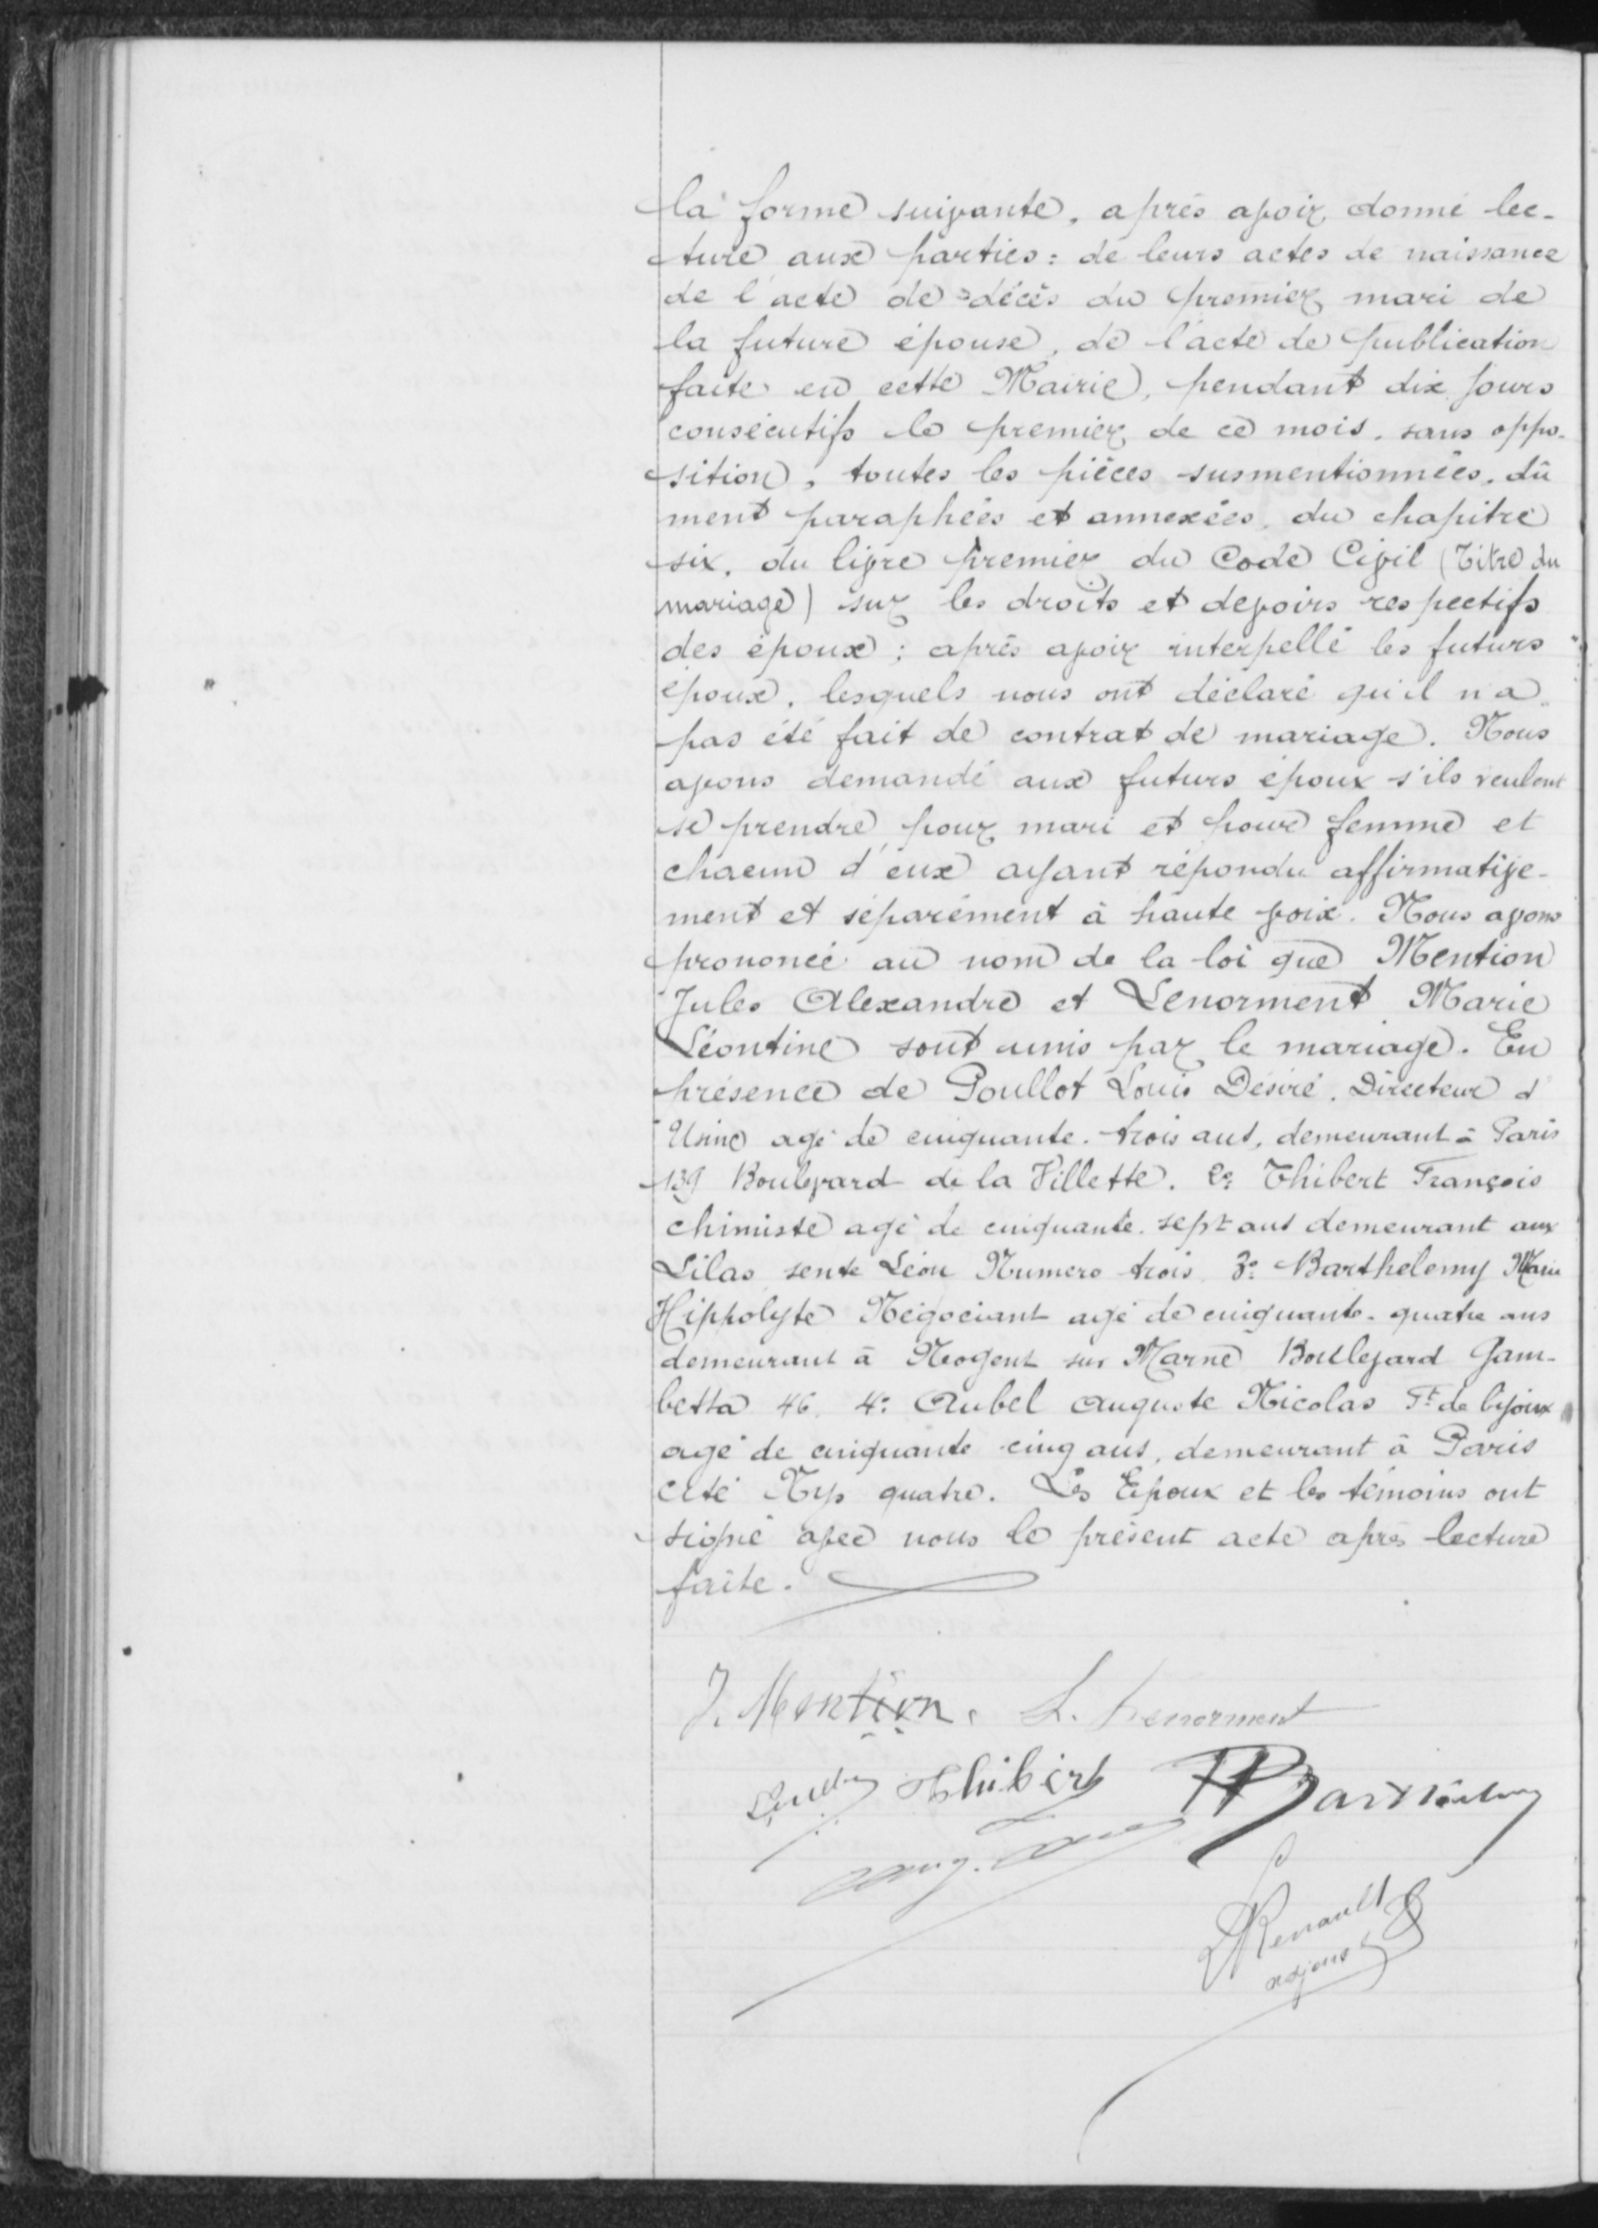la forme suivant, après avoir donné lec-
ture aux parties: de leurs actes de naissance
de l'acte de décès du premier mari de
la future épouse de l'acte de publication
faite en cette Mairie, pendant dix jours
consécutifs le premier de ce mois, sans oppo
sition, toutes les pièces susmentionnées, dû
ment paraphées et annexées du chapitre
six, du livre permier du code Civil (Titre du
mariage) sur les droits et devoirs respectifs
des époux); après avoir interpellé les futurs
époux), lesquels nous ont déclaré qu'il n'a
pas été fait de contrat de mariage. Nous
avons demandé aux futurs époux s'ils veulent
se prendre pour mari et pour emme et
chacun d'eux ayant répondu affirmative-
ment et séparément à haute voix. Nous avons
prononcé au nom de la loi que Mention
Jules Alexandre et Lenorment Marie
Léontine sont unis par le mariage. En
présence de Poullot Louis Désiré. Directeur d'
Usine) agé de cinquante. trois ans, demeurant à Paris
139 Boulevard de la Villette. 2° Thibert François
Chimiste agé de cinquante-sept ans demeurant aux
Lilas sense Léon Numero trois 3° Barthelemy Marie
Hippolyte Négociant agé de cinquante-quatre ans
demeurant à Nogent sur Marne Boulevard Gam-
betta 46 4° Aubel Auguste Nicolas F de bijoux
agè de cinquante cinq ans, demeurant à Paris
cite Mys quatre. Les Epoux et les témoins ont
signé avec nous les présent acte après lecture
faite.
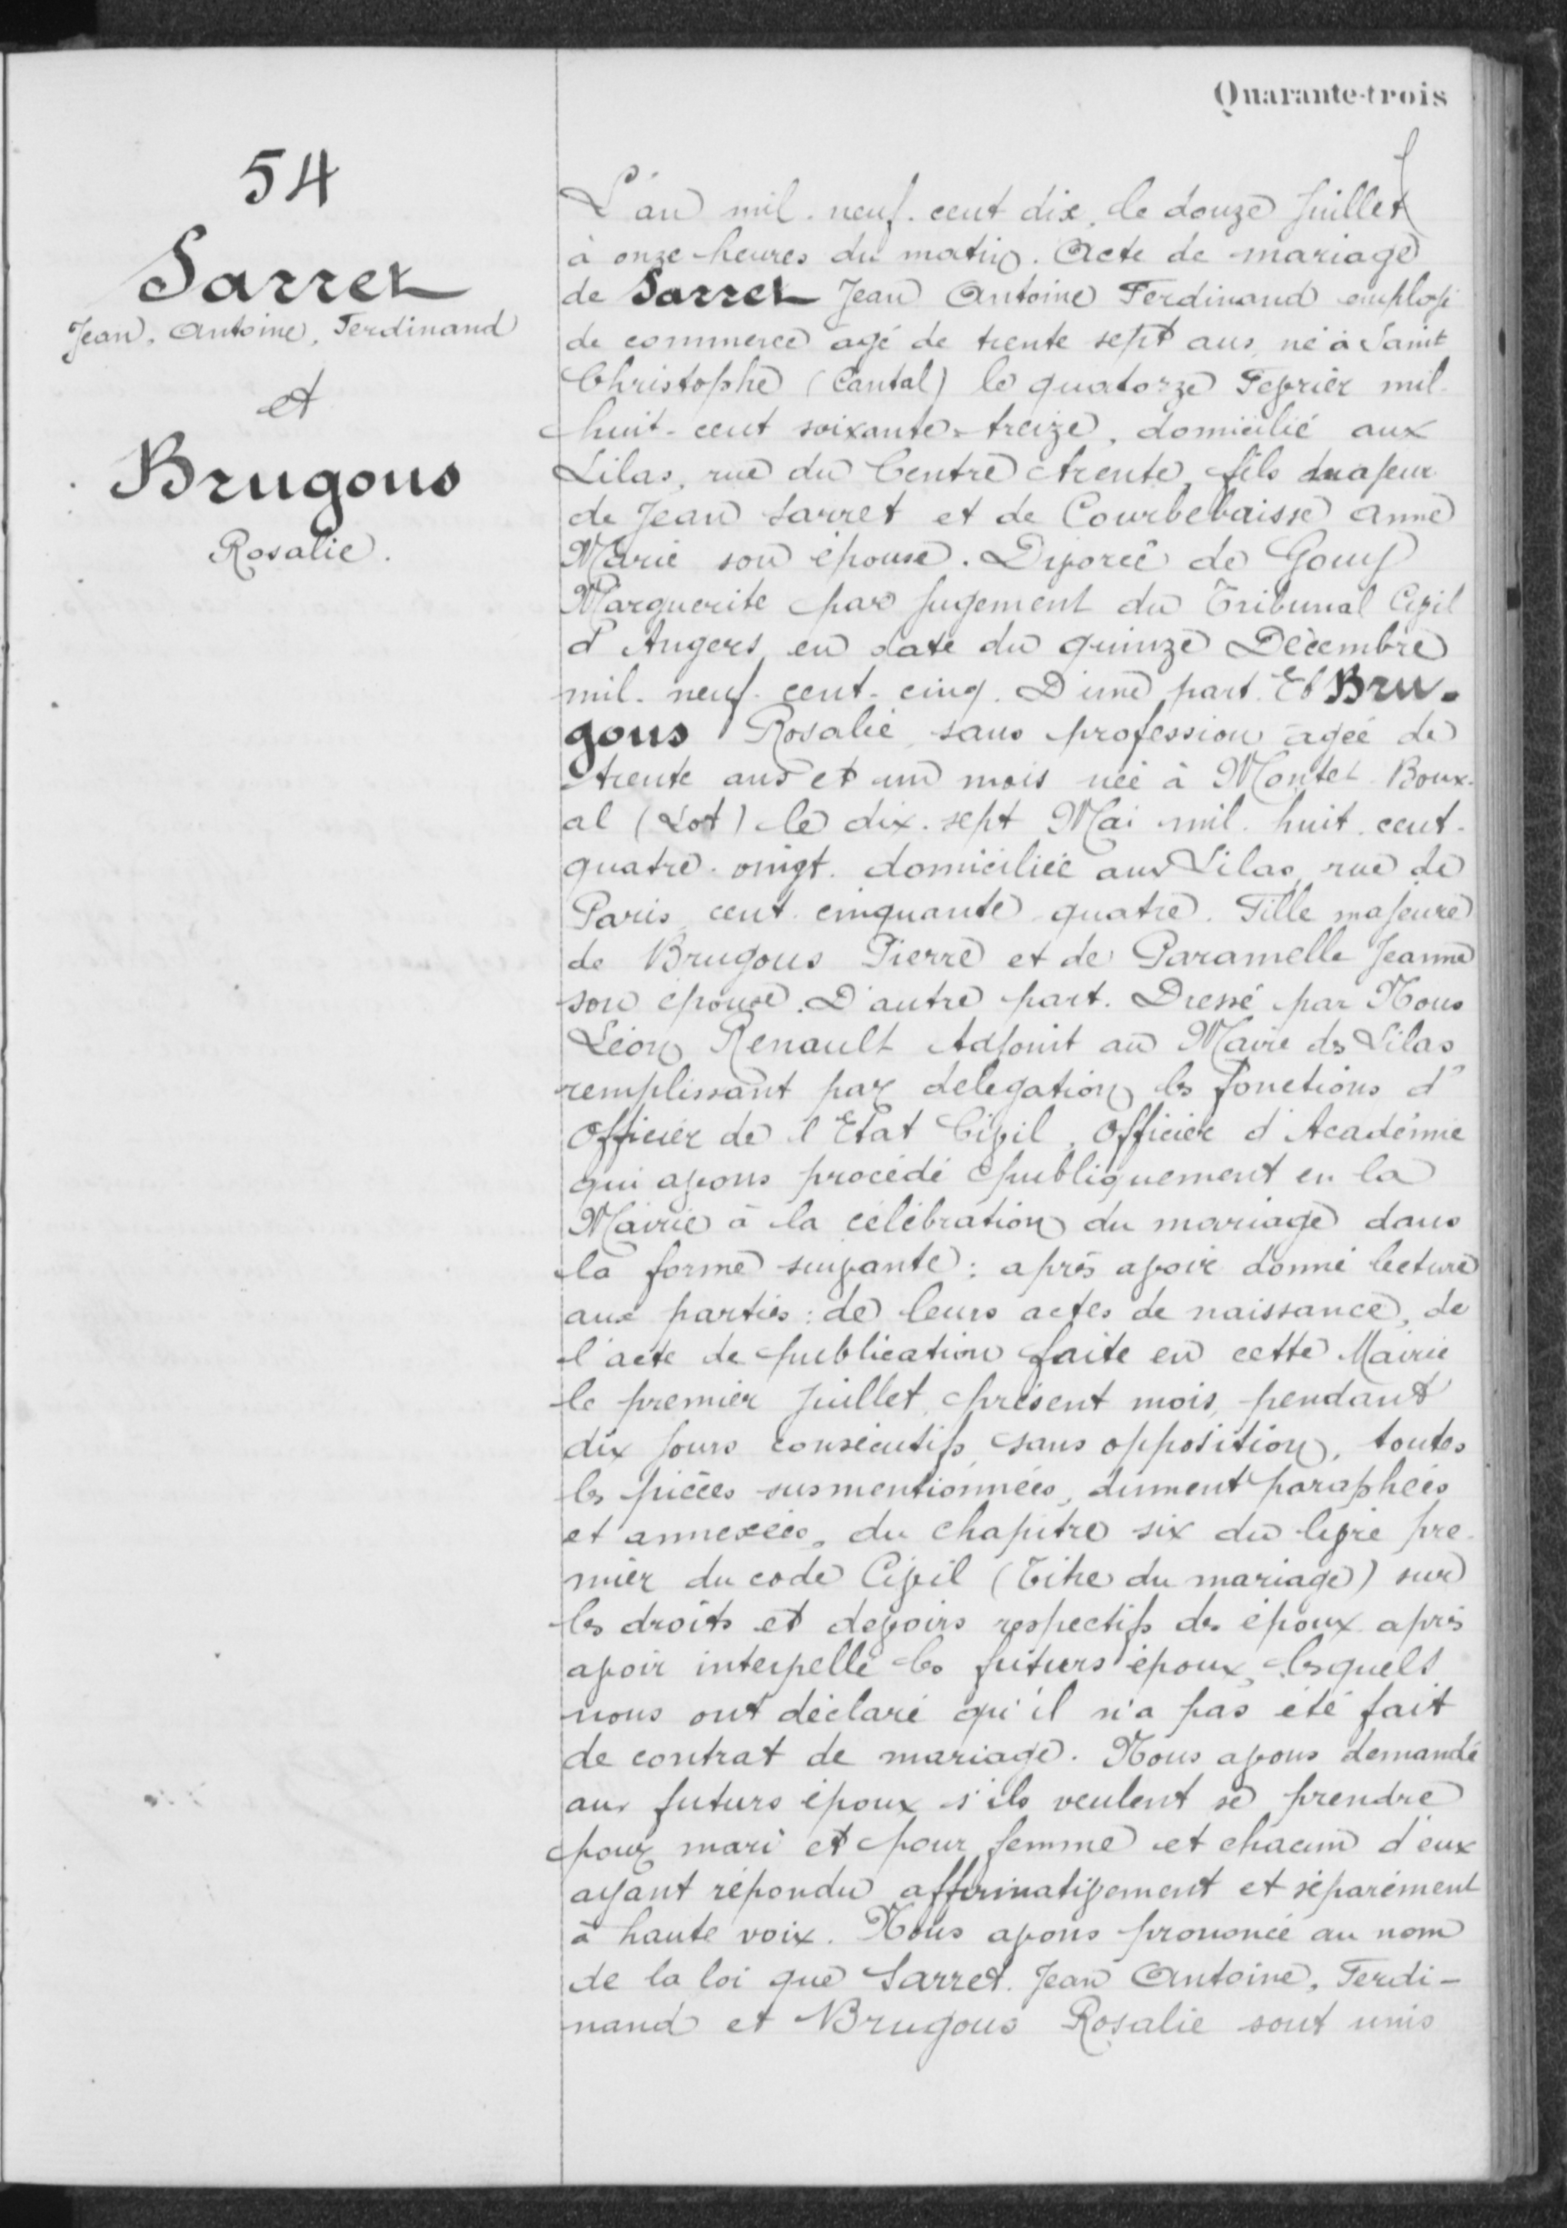54
Sarret
Jean-Antoine, Ferdinand
et
Brugous
Rosalie
L'an mil-neuf cent dix, le douze Juillet
à onze heures du matin. Acte de mariage
de Sarret Jean Antoine Ferdinand employé
de commerce âgé de trente sept ans né à Saint
Christophe (Cantal) le quatorze Fevrier mil
huit-cent soixante treize, domicilié aux
Lilas, rue du Centre trente, fils majeur
de Jean Sarret et de Courbelaisse Anne
Marie son épouse. Divorcé de Gou
Marguerite par Jugement du Tribunal Civil
d'Angers en date du quinze Décembre
mil-neuf-cent-cinq. D'une part. Et Bru-
gous Rosalie, sans profession, âgée de
trente ans et un mois née à Montet Boux)
al (Lot) le dix-sept Mai mil-huit cent
quatre-vingt domiciliée au Lilas rue de
Paris, cent-cinquante-quatre. Fille majeure
de Brugous Pierre et de Paramelle Jeanne
son épouse. D'autre part. Dressé par Nous
Léon Renault Adjoint au Maire des Lilas
remplissant par delegation les fonction d'
Officier de l'Etat Civil; Officier d'Académie
qui avons procédé publiquement en la
Mairie à la célébration du mariage dans
la forme suivante: après avoir donné lecture
aux parties: de leurs actes de naissance, de
l'acte des publication faite en cette Mairie
le premier Juillet présent mois, pendant
dixjours consécutifs, sans oppositions, toutes
les pièces susmentionnées, dûment paraphées
et annexées. du chapitro six du livre pre-
mier du code Civil (Titre du mariage) sur
les droits et devoirs respectifs des époux après
avoir interpellé les futurs époux, lesquels
nous ont déclaré qu'il n'a pas été fait
de contrat de mariage. Nous avons demandé
aux futurs époux s'ils veulent se prendre
pour mari et pour femme et chacun d'eux
ayant répondu affirmativement et séparément
à haute voix. Nous avons prononcé au nom
de la loi que Sarret Jean Antoine, Ferdi-
nand et Brugous Rosalie sont unis